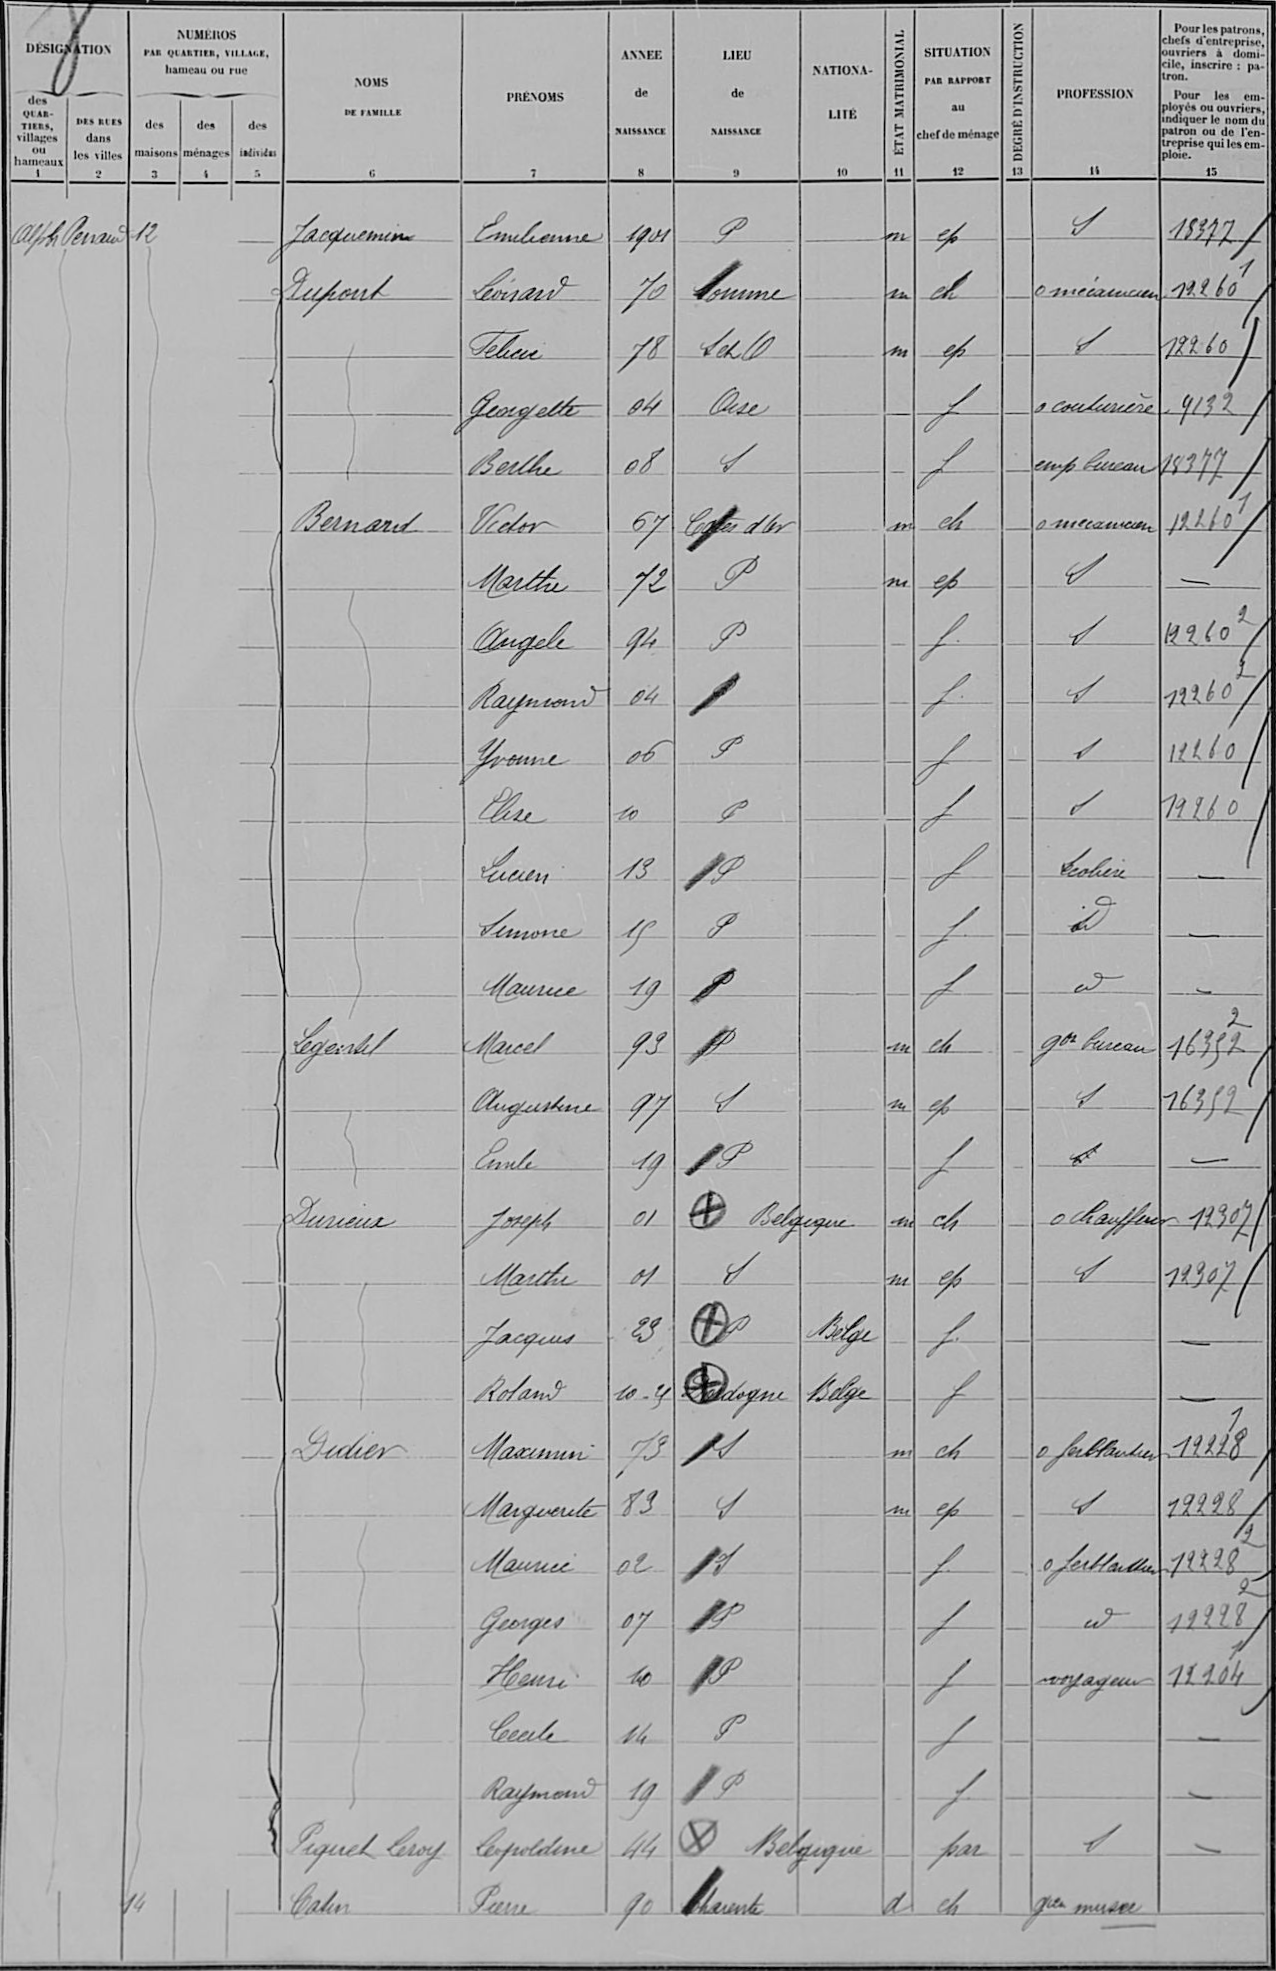Jacquemin/Emilienne/1901/P/¤/m/ep/¤/S/18377
Dupont/Levinard/70/Somme/¤/m/ch/¤/o mécanicien/12260
¤/Felicie/78/S et O/¤/m/ep/¤/S/12260
¤/Georgette/04/Oise/¤/¤/f/¤/o couturière/9132
¤/Berthe/08/S/¤/¤/f/¤/emp bureau/18377
Bernard/Victor/67/Cotes d'Or/¤/m/ch/¤/o mecanicien/12260
¤/Marthe/72/P/¤/m/ep/¤/S/¤
¤/Angele/94/P/¤/¤/f/¤/S/12260
¤/Raymond/04/P/¤/¤/f/¤/S/12260
¤/Yvonne/06/P/¤/¤/f/¤/S/12260
¤/Elise/10/P/¤/¤/f/¤/S/12260
¤/Lucien/13/P/¤/¤/f/¤/ecoliere/¤
¤/Simone/15/P/¤/¤/f/¤/id/¤
¤/Maurice/19/P/¤/¤/f/¤/id/¤
Legenlil/Marcel/93/P/¤/m/ch/¤/gon bureau/16352
¤/Augustine/97/S/¤/m/ep/¤/S/16352
¤/Emile/19/P/¤/¤/f/¤/S/¤
Durieux/Joseph/01/Belgique/¤/m/ch/¤/o chauffeur/12307
¤/Marthe/01/S/¤/m/ep/¤/S/12307
¤/Jacques/29/P/Belge/¤/f/¤/¤/¤
¤/Roland/10 21/Dordogne/Belge/¤/f/¤/¤/¤
Didier/Maximin/73/S/¤/m/ch/¤/o ferblantier/12228
¤/Marguerite/89/S/¤/m/ep/¤/S/12228
¤/Maurice/02/S/¤/¤/f/¤/o ferblantier/12228
¤/Georges/07/P/¤/¤/f/¤/id/12228
¤/Henri/10/P/¤/¤/f/¤/voyageur/12204
¤/Cecile/14/P/¤/¤/f/¤/¤/¤
¤/Raymond/19/P/¤/¤/f/¤/¤/¤
Piquel Leroy/Leopoldine/44/Belgique/¤/¤/Par/¤/S/¤
Calin/Pierre/90/Charente/¤/d/ch/¤/gien musee/¤
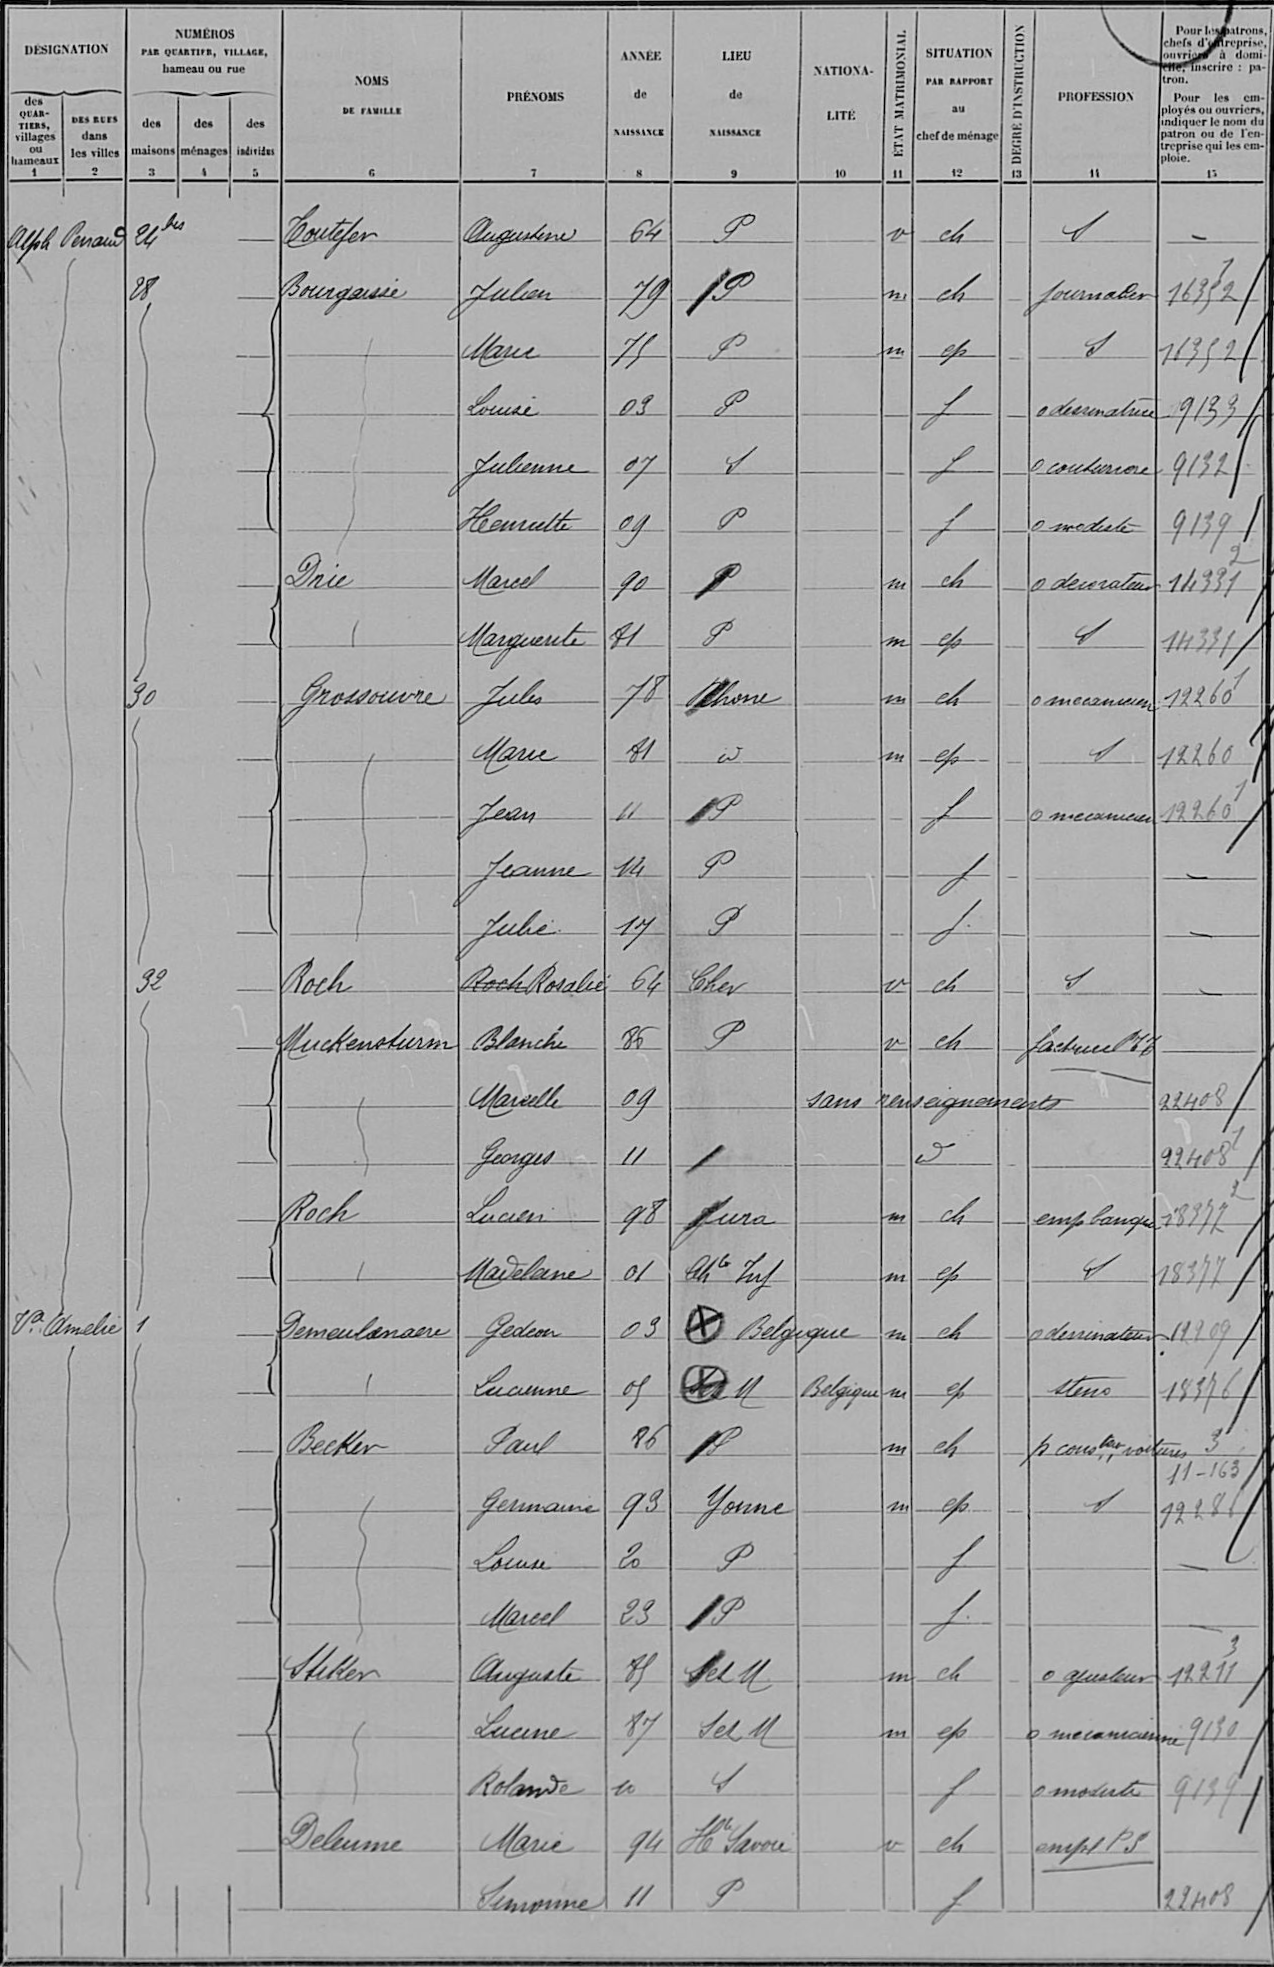Coutefer/Augustine/64/P/¤/v/ch/¤/S/¤
Bourgaisie/Julien/79/P/¤/m/ch/¤/journalier/16352
¤/Marie/75/P/¤/m/ep/¤/S/16352
¤/Louise/03/P/¤/¤/f/¤/o dessinatrice/9133
¤/Julienne/07/S/¤/¤/f/¤/o couturiere/9132
¤/Henriette/09/P/¤/¤/f/¤/o modiste/9139
Drie/Marcel/90/P/¤/m/ch/¤/o decorateur/14331
¤/Marguerite/81/P/¤/m/ep/¤/S/14331
Grossouvre/Jules/78/Rhone/¤/M/ch/¤/o mecanicien/12260
¤/Marie/81/id/¤/m/ep/¤/S/12260
¤/Jean/11/P/¤/¤/f/¤/o mecanicien/12260
¤/Jeanne/14/P/¤/¤/f/¤/¤/¤
¤/Julie/17/P/¤/¤/f/¤/¤/¤
Roch/RochRosalie/64/Cher/¤/V/ch/¤/S/¤
Muckenshurm/Blanche/86/P/¤/V/ch/¤/facteur PTT/¤
¤/Marielle/09/¤/sans renseignements/¤/¤/¤/¤/22408
¤/Georges/11/¤/¤/¤/id/¤/¤/22408
Roch/Lucien/98/Jura/¤/m/ch/¤/emp banque/18377
¤/Madeleine/01/Chte Inf/¤/m/ep/¤/S/18377
Demeulenaere/Gedeon/09/Belgique/¤/m/ch/¤/o dessinateur/19909
¤/Lucienne/05/S et M/Belgique/m/ep/¤/steno/18376
Becker/Paul/86/P/¤/m/ch/¤/p construt voitures/3!11 163
¤/Germaine/93/Yonne/¤/m/ep/¤/S/12286
¤/Louise/20/P/¤/¤/f/¤/¤/¤
¤/Marcel/23/P/¤/¤/f/¤/¤/¤
Slicker/Auguste/85/s et m/¤/m/ch/¤/o ajusteur/12211
¤/Lucine/87/s et m/¤/m/ep/¤/o mecanicienne/9130
¤/Rolande/10/S/¤/¤/f/¤/o modiste/9139
Deleume/Marie/94/H Savoie/¤/V/ch/¤/empl PS/¤
¤/Simonne/11/P/¤/¤/f/¤/¤/22408